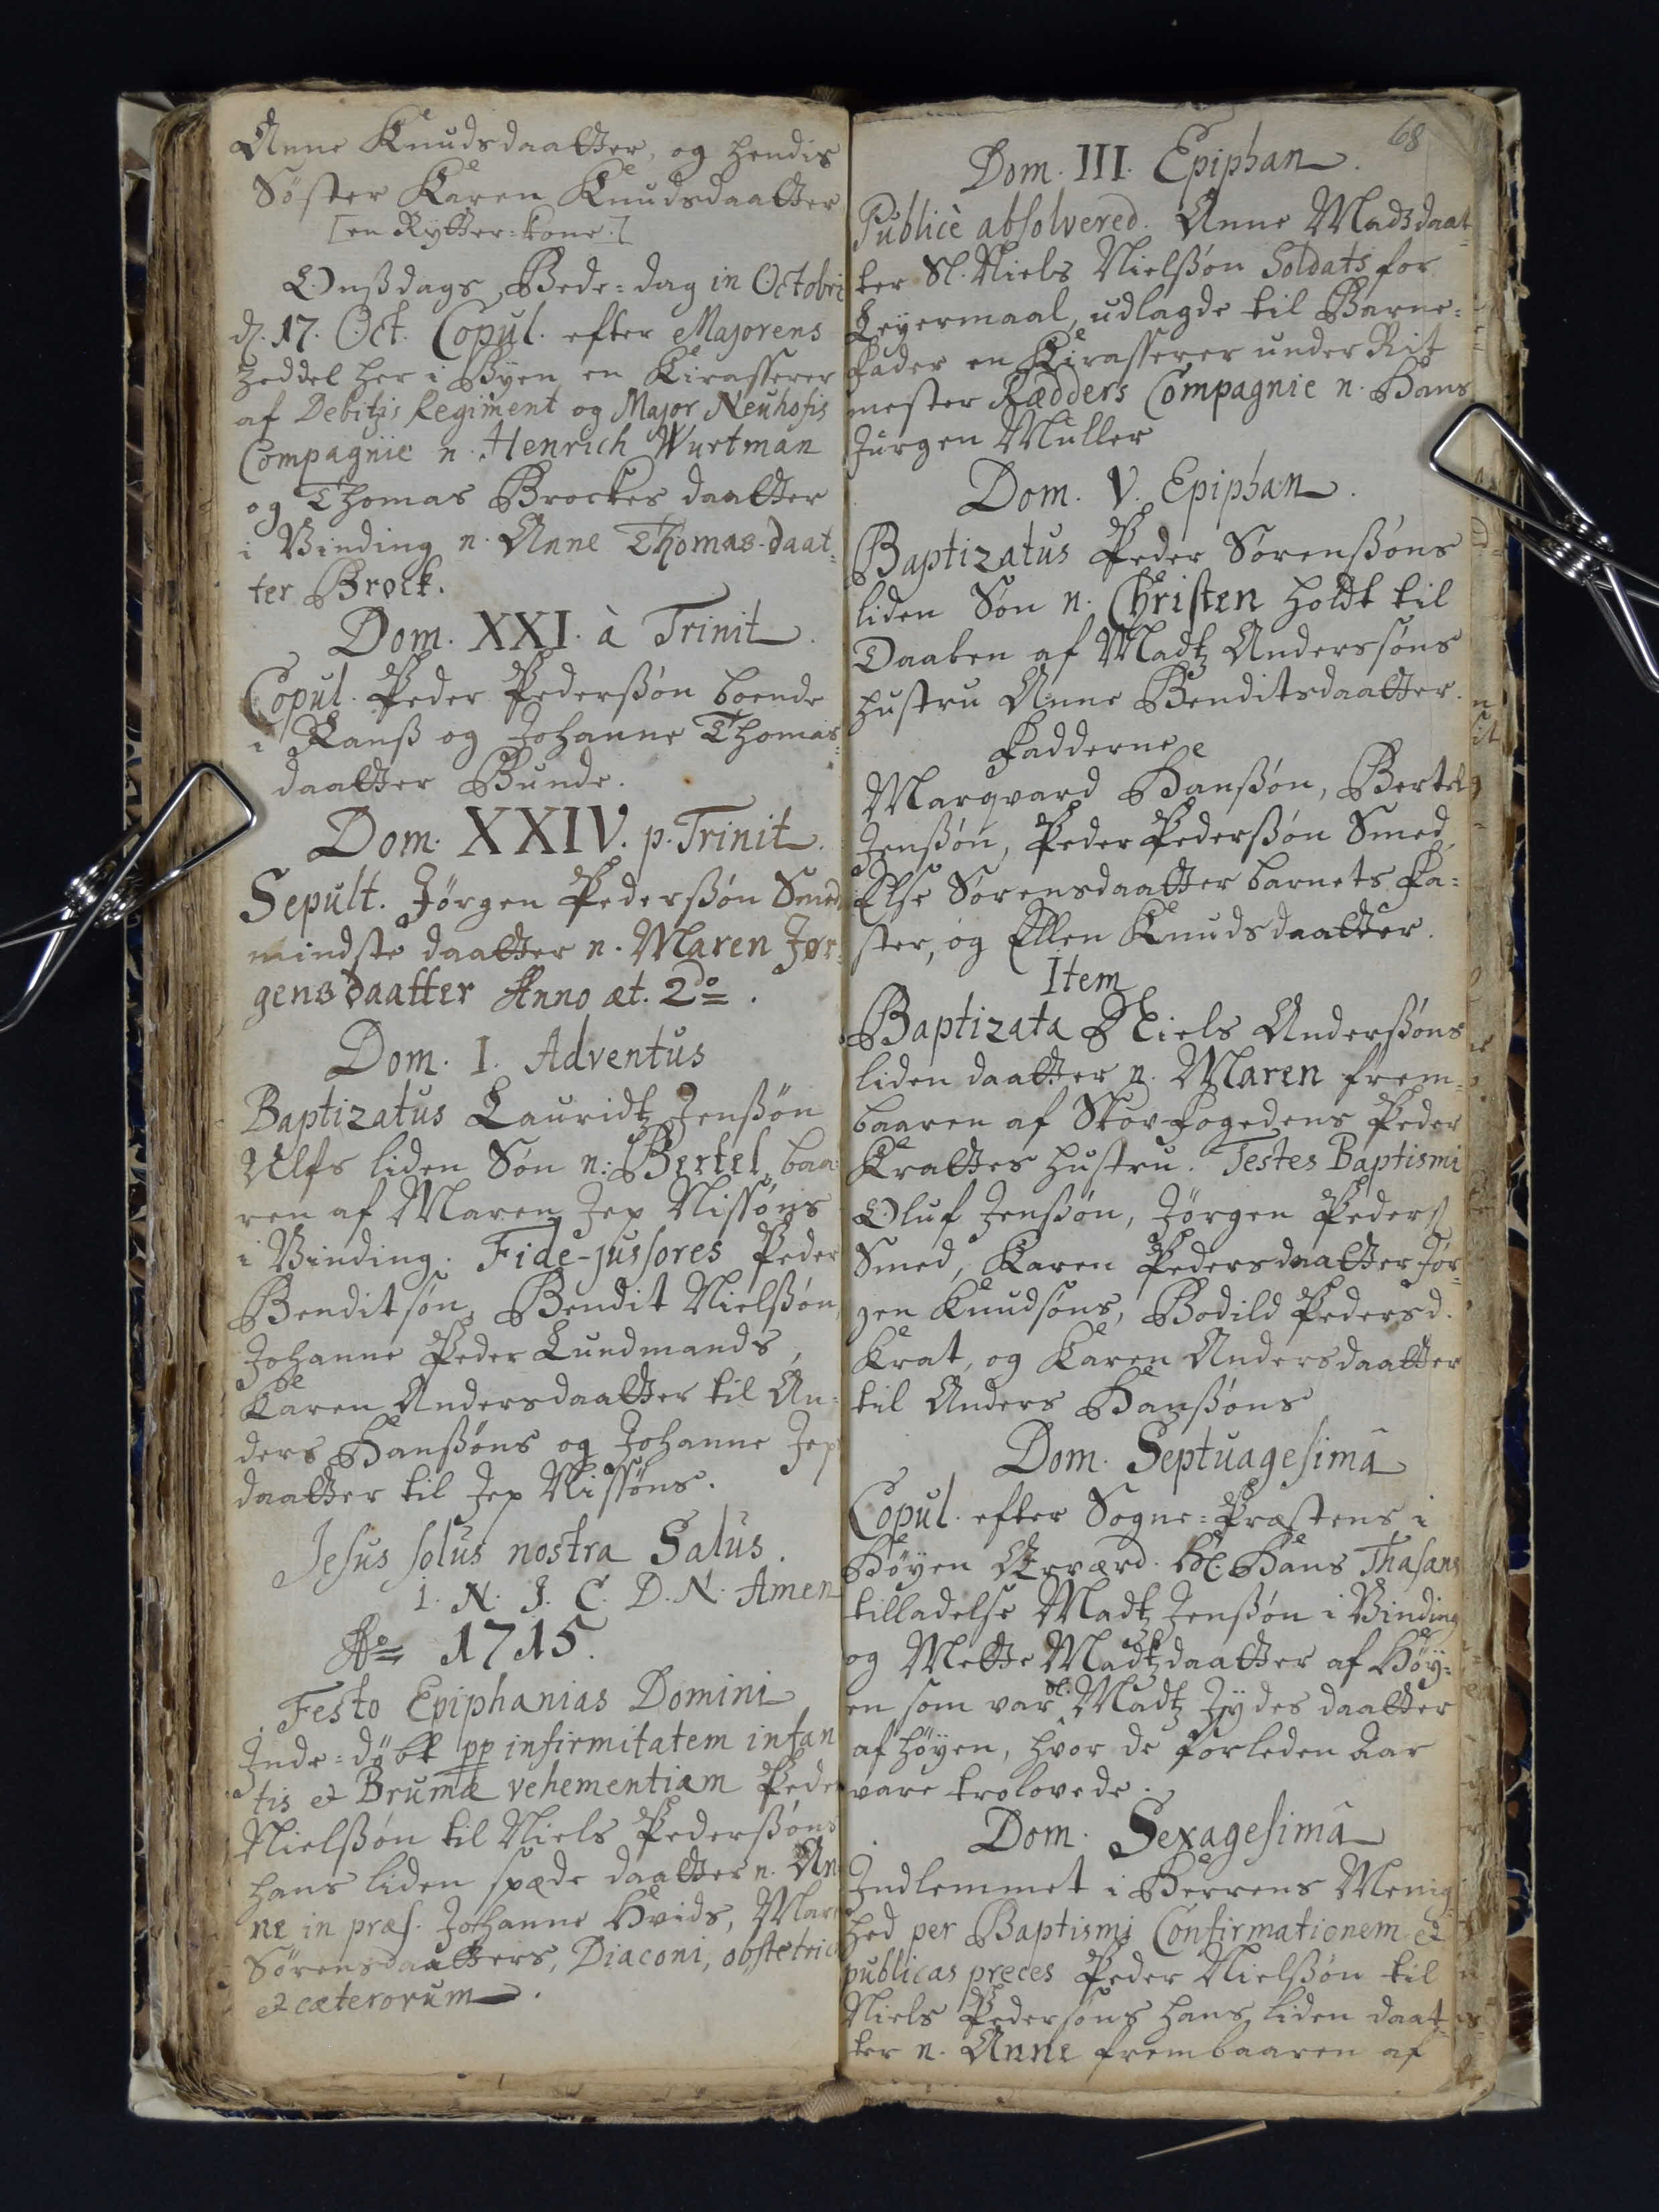Anne Knudsdaatter, og hendis
Søster Karen Knudsdaatter 
[en Rytter=kone.]
Onssdags Bede=dag in Octobri
d. 17. Oct. Copul. efter Majorens
Zeddel her i Byen en Kirasserer
af Debitzis Regiment og Major Neuhofis 
Compagnie n. Henrich Wurtman
og Thomas Brockes daatter
i Vinding n. Anne Thomas-daat¬
ter Brock.
Dom. XXI. a Trinit
Copul. Peder Pederssøn boende
i Ranss og Johanne Thomas¬
daatter Bunde.
Dom. XXIV. p. Trinit
Sepult. Jørgen Pederssøn Smed 
mindste daatter n. Maren Jør¬
gensdaatter Anno æt. 2do
Dom. I. Adventus
Baptizatus Lauridtz Jenssøn
Ulfs liden Søn n: Bertel, baa¬
ren af Maren Jep Nissøns
i Vinding. Fide-jusores Peder
Benditsøn,  Bendit Nielssøn,
Johanne Peder Lundmands
Karen Andersdaatter til An¬
ders Hanssøns og Johanne Jep 
daatter til Jep Nissøns.
Jesus solus nostra Satus
I. N. J. C. D. N. Amen
Ao 1715
Festo Epiphanias Domini
Inde=døbt pp infirmitatem infan
tis et Brumæ vehementiam Peder
Nielssøn til Niels Pederssøns
hans liden spæde daatter n. An
ne, in præs. Johanne Hvids, Mare 
Sørensdaatters, Diaconi, obstetric 
et cæterorum.
68
Dom. III Epiphan
Publice absolvered Anne Madsdaat¬
ter Sl. Niels Nielssøn Soldats for
Leyermaal, udlagde til Barne=
Fader en Kirasserer under Rit
mester Rædders Compagnie n. Hans
Jurgen Muller
Dom. V. Epiphan.
Baptizatus Peder Sørenssøns
liden Søn n. Christen holdt til
Daaben af Madtz Anderssøns
hustru Anne Benditsdaatter.
Fadderne
Marqvard Hanssøn, Bertel
Jenssøn, Peder Pederssøn Smed
Else Sørensdaatter barnets Fa¬
ster, og Ellen Knudsdaatter
Item
Baptizata Niels Anderssøns
liden daatter n. Maren frem¬
baaren af Skov-Fogedens Peder
Krattes hustru. Testes Baptimi
Oluf Jenssøn, Jørgen Peders
Smed, Karen Pedersdaatter Jør¬
gen Knudsøns, Bodild Pedersd.
Krat, og Karen Andersdaatter
til Anders Hanssøns
Dom. Septuagesima
Copul. efter Sogne=Præstens i
Høyen Vrrærd. Hl Hans Thasans
tilladelse Madtz Jenssøn i Vinding
og Mette Madtzdaatter af Høy¬
Sl.
en som var Madtz Jydes daatter
af Høyen, hvor de forleden Aar
vare trolovede
Dom. Sexagesima
Indlemmet i Herrens Menig
hed per Baptismi Confirmationem et 
publicas preces Peder Nielssøn til
Niels Pedersøns hans liden daat¬
ter n. Anne, frembaaren af